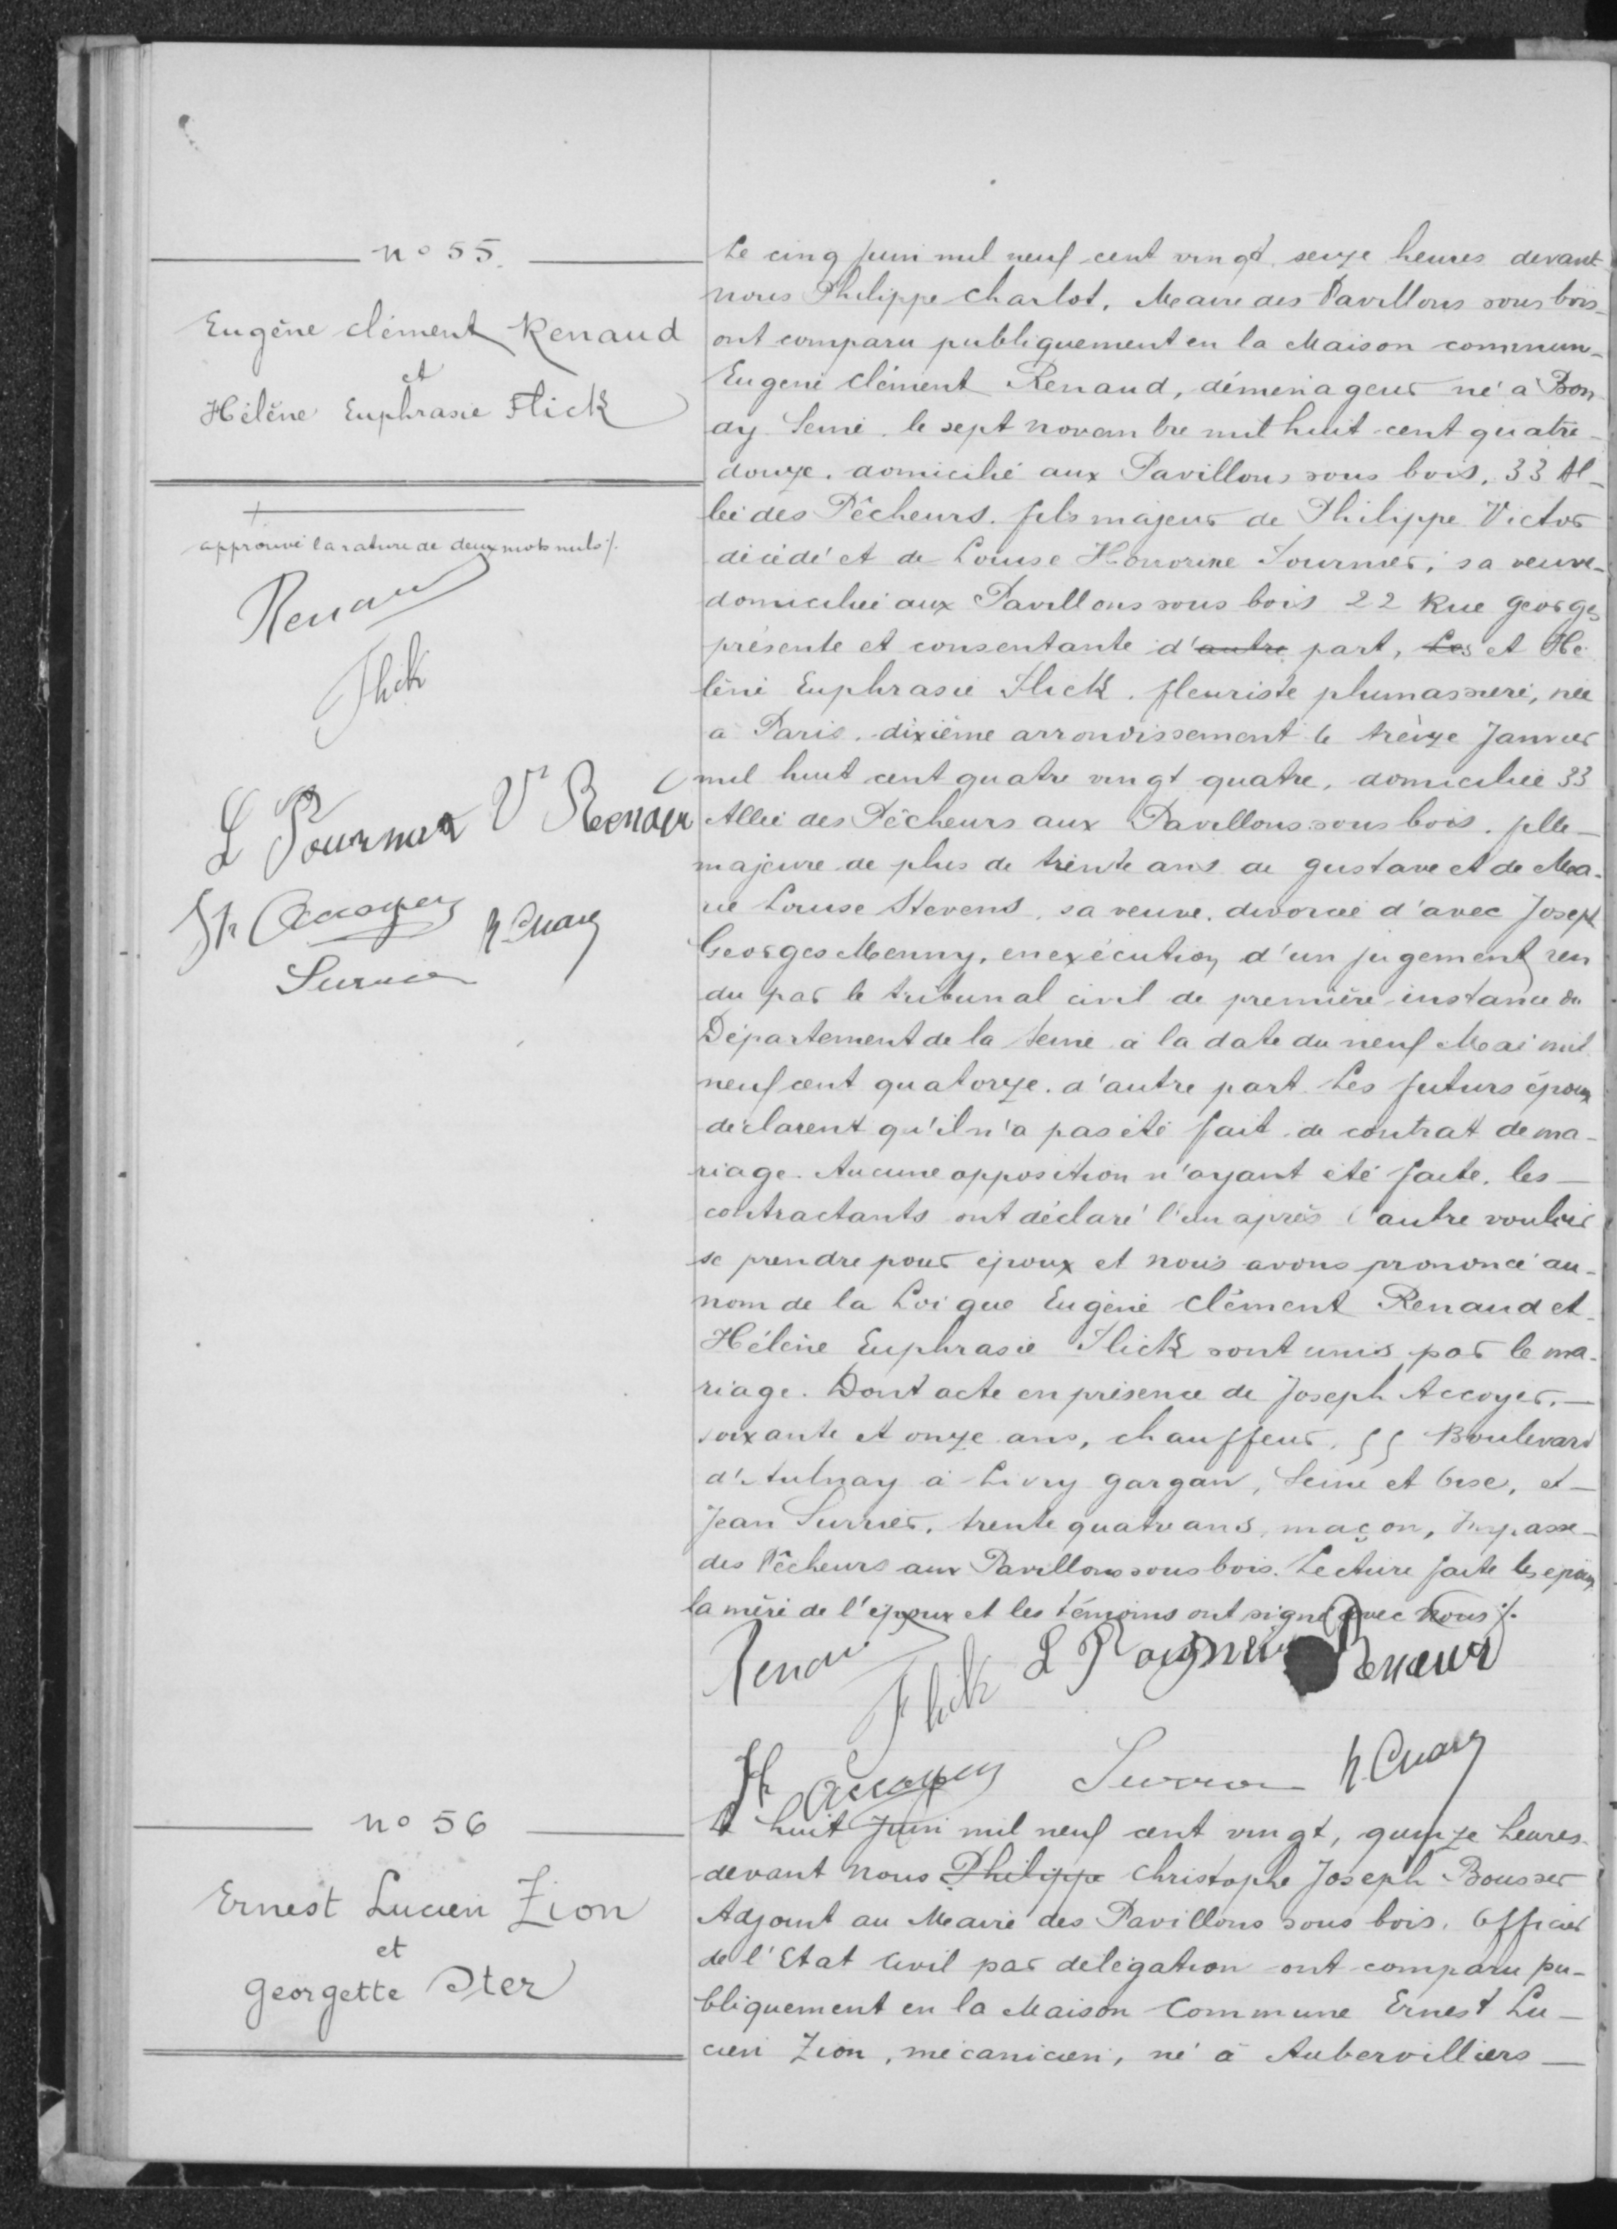N°55
Eugène Clément Renaud
et
Hélène Euphrasie Flick
Le cinq Juin mil neuf cent vingt seize heures devant
nous Philippe Charlot, Maire des Pavillons sous bois
ont comparu publiquement en la Maion commune
Eugène Clément Renaud, déménageur né à Bon
ay Seine le sept novembre mil huit cent quatre
douze, domicilié aux Pavillons sous bois, 33 Al-
lée des Pêcheurs fils majeur de Philippe Victor
décédé et de Louise Honorine Journier, sa veuve
domiciliée aux Pavillons sous bois 22 Rue Georges
présente et consentante d'autre part, Les et Hé
lène euphrasie Flick, fleuriste plumassiere, née
à Paris, dixième arrondissement le treize Janvier
mil huit cent quatre vingt quatre, domiciliée 33
Allée des Pêcheurs aux Pavillons sous bois, fille
majeure de plus de trente ans de gustave et de Ma
rie Mouise Stevens, sa veuve, divorcée d'avec Joseph
Georges Menny, en exécution d'un jugement ren
du par le tribunal civil de première instance du
Département de la Seine à la date du neuf Mai mil
neuf cent quatorze d'autre pat. Les futurs époux
déclarent qu'il n'a pas été fait de contrat de ma-
riage. Aucune opposition n'ayant été faite, les
contractants ont déclaré l'un après l'autre vouloir
se prendre pour époux et nous avons prononcé au
nom de la Loi que Eugène Clément Renaud et
Hélène Euphrasie Flick sont unis par le ma
riage. Dont acte en présence de Joseph Accoyer.
soixante et onze ans, chauffeur, 55 Boulevard
à Sulnay à Livry Gargan, Seine et Oise, et
Jean Surrier, trente quatre ans, maçon, Impasse
des Pêcheurs aux Pavillons sous bois. Lecture faite les époux
la mère de l'époux et les témoins ont signé avec nous ./.
N°56
Ernest Lucien Zion
et
Georgette Oter
Le huit Juin mi neuf cent vingt, quinze heures
devant nous Philippe Christophe Joseph Bousser
Adjoint au Maire des Pavillons sous bois, officier
de l'Etat Civil par délégation ont comparu pu-
bliquement en la Maison Commune Ernest Lu-
cien Zion, mécanicien, né à Aubervilliers-
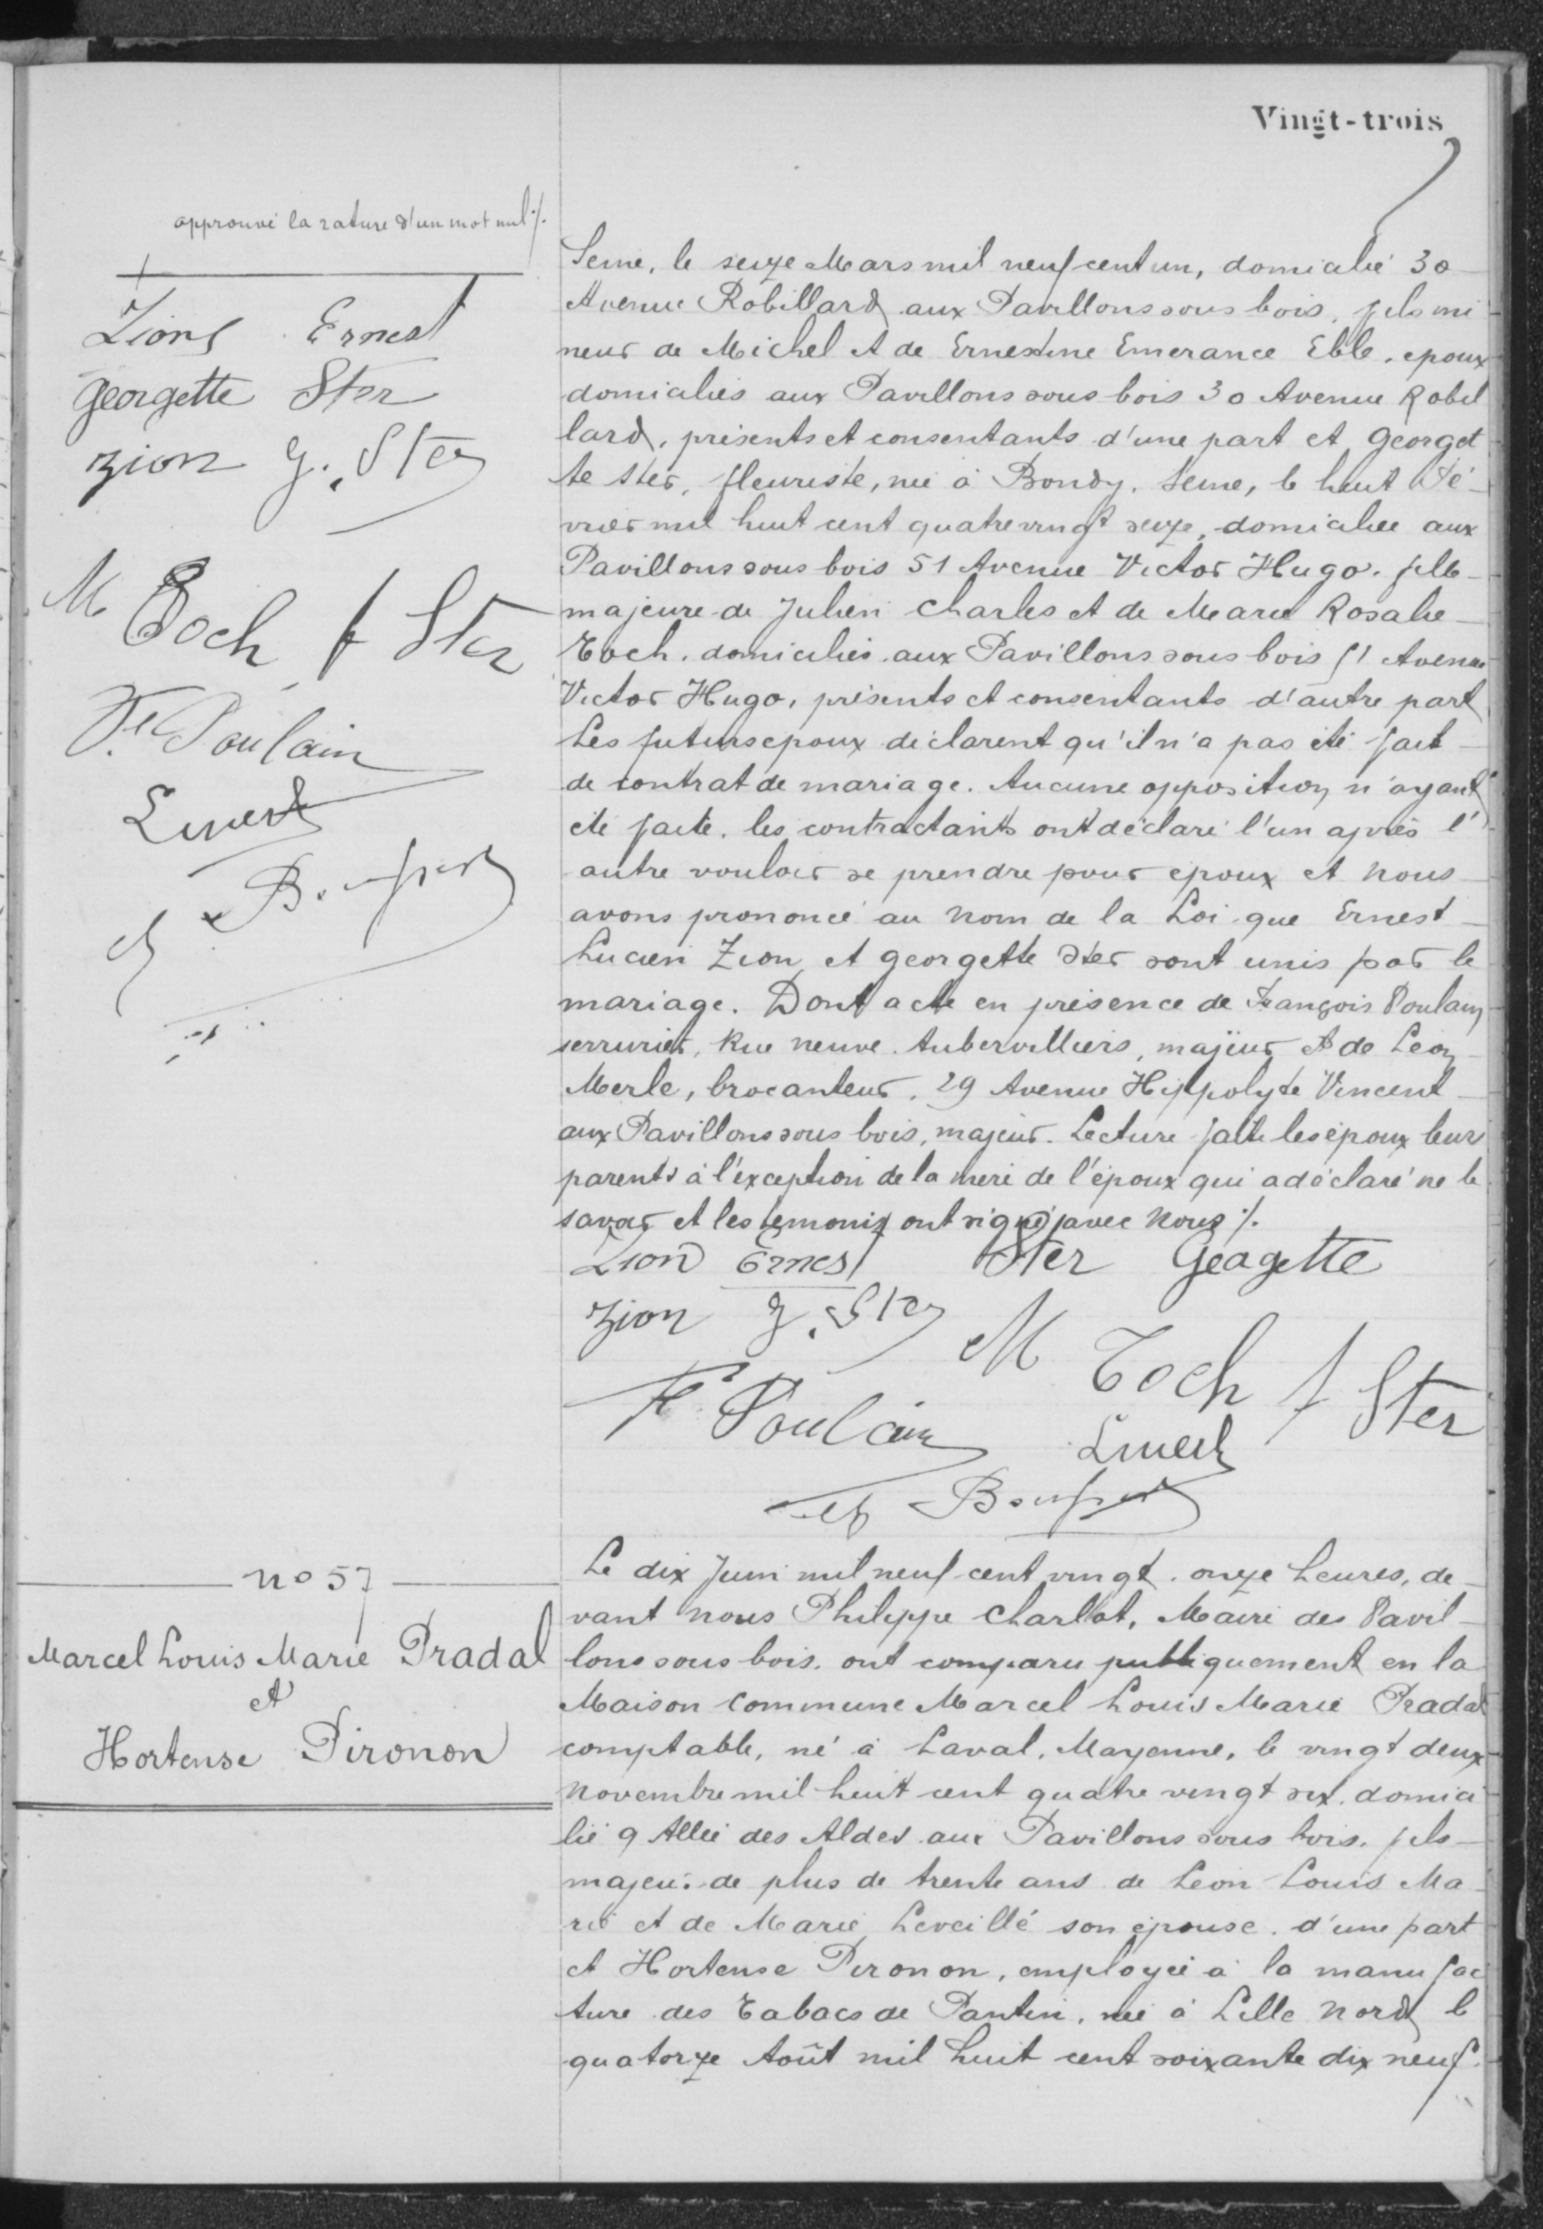Seine, le seize Mars mil neuf cent un, domicilié 30
Avenue Robillard aux Pavillons sous bois, fils mi
neur de Michel et de Ernestine Emerance Eble, epoux
domiciliés aux Pavillons sous bois 30 Avenue Robil
lard, présents et consentants d'une part et Georgot
te Alex, fleuriste, née à Boudy, seine, le huit Fé-
vrier mil huit cent quatre vingt seize, domiciliée aux
Pavillons sous bois 51 Avenue Victor Hugo, fille
majeure de Julien Charles et de Marie Rosalie
Roch, domiciliés aux Pavillons sous bois 51 Avenue
Victor Hugo, présents et consentants d'autre part
Les futurs epoux déclarent qu'il n'a pas été fait
de contrat de mariage. Aucune opposition n'ayant
été faite, les contractants ont déclaré l'un après l'
autre vouloir se prendre pour epoux et nous
avons prononcé au nom de la Loi que Ernest
Lucien Zion et Georgette Oter sont unis par le
mariage. Dont acte en présence de François Poulam
serrurier, Rue neuve, Aubervilliers, majeur et de Leon
Merle, brocanteur, 29 Avenue Hippolyte Vincent
aux Pavillons sous bois, majeur. Lecture faite les époux leur
parents à l'exception de la mère de l'époux qui a déclaré ne le
savoir et les témoins ont signé avec nous ./.
N°57
Marcel Louis Marie Pradal
et
Hortense Pironon
Le dix Juin mil neuf cent vingt onze heures, de-
vant nous Philipe Charlot, Maire des Pavil-
lons sous bois ont comparu publiquement en la
Maison commune Marcel Louis Marie Pradat
comptable, né à Laval, Mayenne, le vingt deux
novembre mil huit cent quatre vingt six domici
lié 9 Allée des Aldes aux Pavillons sous bois, fils
majeur de plus de trente ans de Léon Louis Ma
rie et de Marie Leveillé son épouse d'une part
et Hortense Pironon, employée à la manufac
ture des tabacs de Pantin, née à Lille nord le
quatorze Août mil huit cent soixante dix neuf